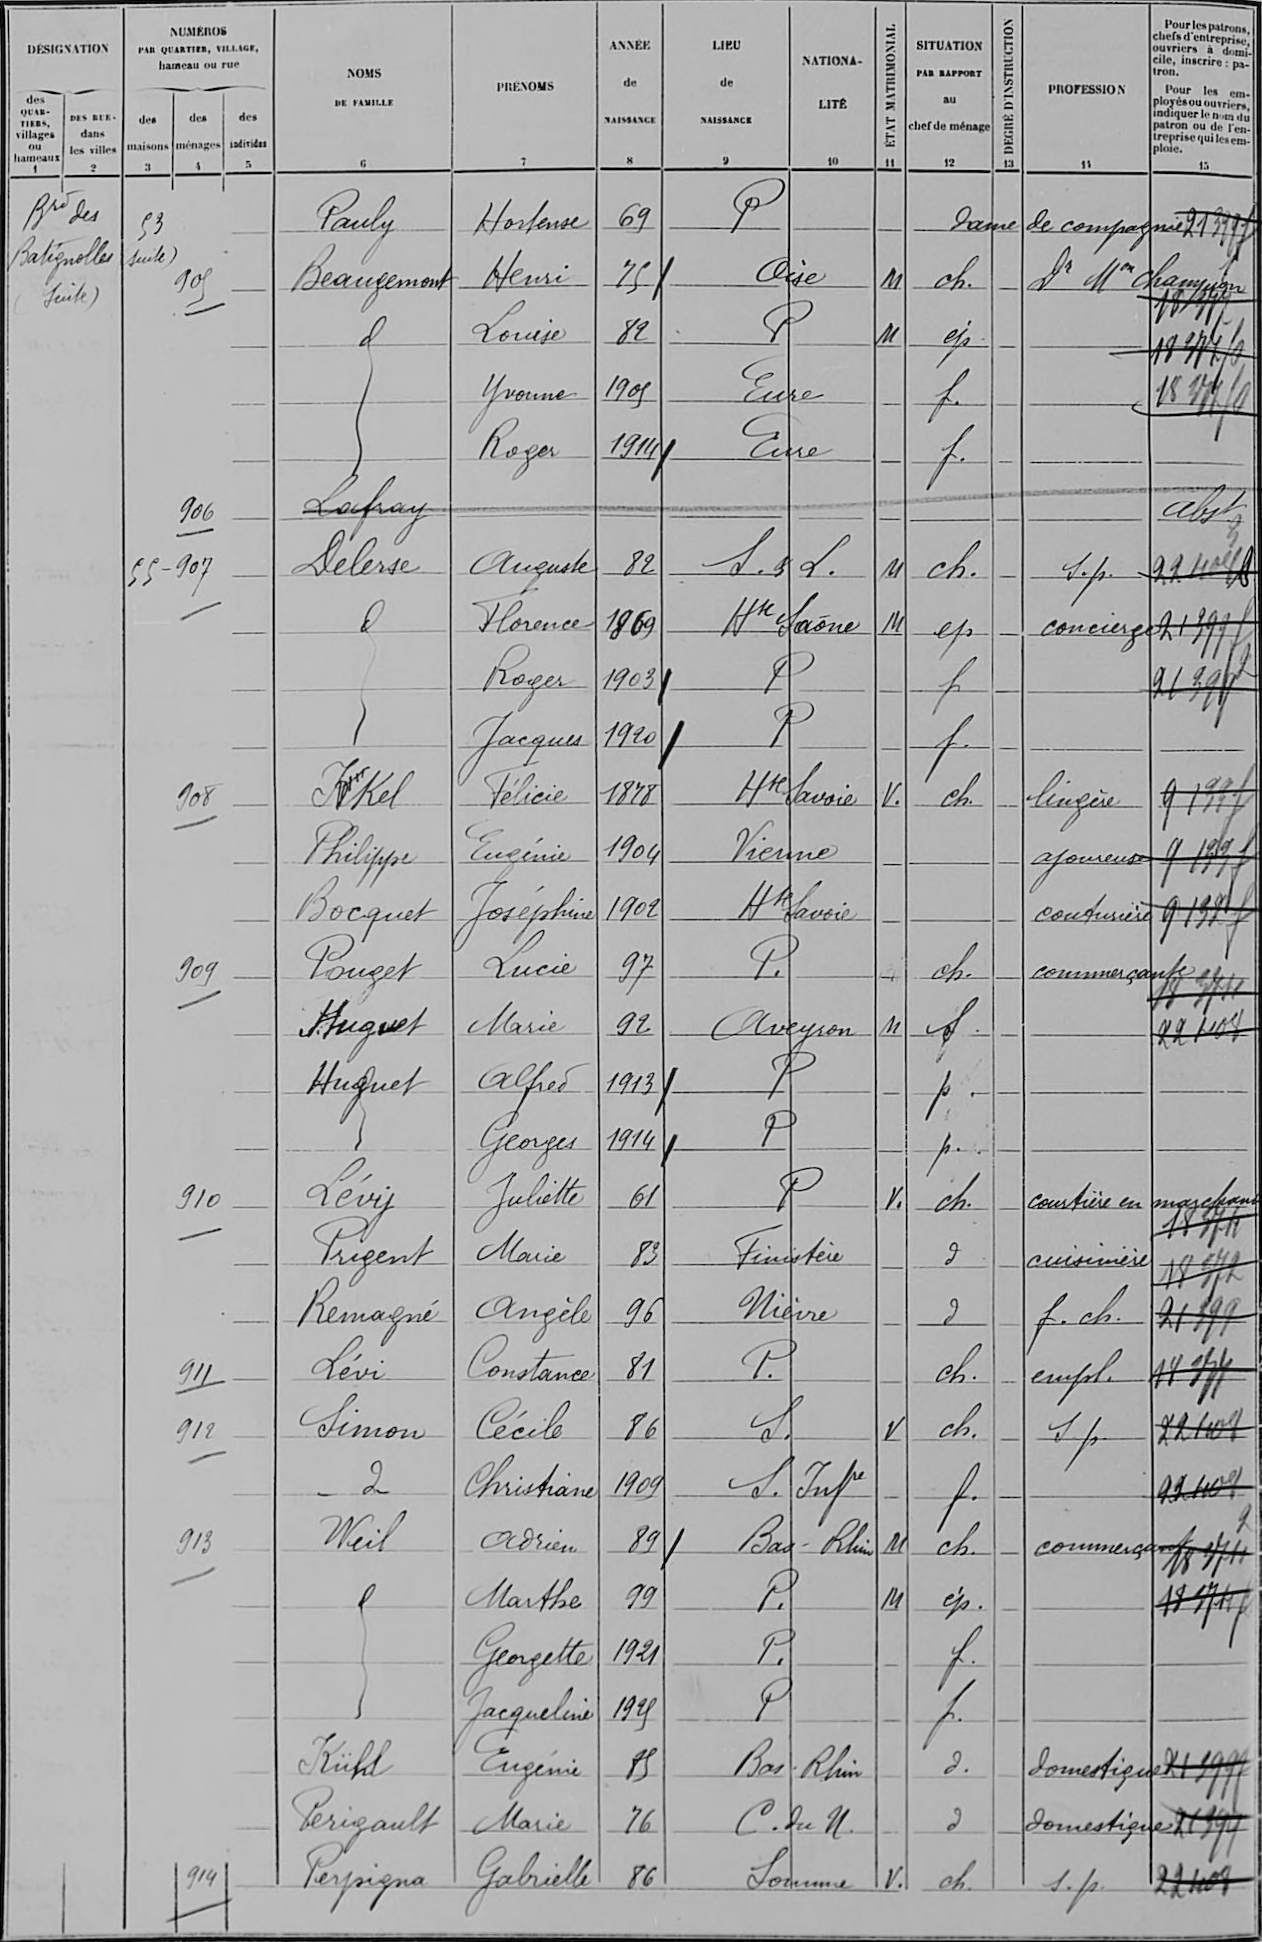Pauly/Hortense/69/P/¤/¤/¤/¤/dame de compagnie/21399 f
Beaugemont/Henri/75/Oise/¤/M/ch /¤/Dr Mon champion/¤
d/Louise/82/P/¤/M/ép/¤/¤/18377 fs
d/Yvonne/1905/Eure/¤/¤/f /¤/¤/18377 fs
d/Roger/1914/Eure/¤/¤/f /¤/¤/¤
Lefray/¤/¤/¤/¤/¤/¤/¤/¤/abs
Delerse/Auguste/82/S & L /¤/M/ch /¤/S p /22408 f
d/Florence/1869/Hte Saône/¤/M/ep/¤/concierge/21399 f
d/Roger/1903/P/¤/¤/f/¤/¤/21399
d/Jacques/1920/P/¤/¤/f /¤/¤/¤
Ikel/Félicie/1878/Hte Savoie/¤/V /ch /¤/lingère/9133 f
Philippe/Eugénie/1904/Vienne/¤/¤/¤/¤/ajoureuse/9133 f
Bocquet/Joséphine/1902/Hte Savoie/¤/¤/¤/¤/couturière/9132 f
Pouget/Lucie/97/P /¤/¤/ch /¤/commerçant/!18374
Huguet/Marie/92/Aveyron/¤/M/f/¤/¤/22408
Huguet/Alfred/1913/P/¤/¤/p /¤/¤/¤
¤/Georges/1914/P/¤/¤/p /¤/¤/¤
Lévy/Juliette/61/P/¤/V /ch /¤/courtière en marchand /!18374
Prigent/Marie/83/Finistère/¤/¤/d/¤/cuisinière/18372
Remagné/Angèle/96/Nièvre/¤/¤/d/¤/f ch /21399
Lévi/Constance/81/P /¤/¤/ch /¤/empl /18377
Simon/Cécile/86/S /¤/V/ch /¤/S p/22408
d°/Chistiane/1909/S Infre/¤/¤/f /¤/¤/22408
Weil/Adrien/89/Bas-Rhin/¤/M/ch /¤/commerçant/18374
d/Marthe/00/P /¤/M/ép/¤/¤/18374
d/Georgette/1921/P /¤/¤/f /¤/¤/¤
d/Jacqueline/1925/P/¤/¤/f /¤/¤/¤
Kühl/Eugénie/85/Bas-Rhin/¤/¤/d /¤/domestique/21399 f
Perigault/Marie/76/C du N /¤/¤/d/¤/domestique/21399
Perpigna/Gabrielle/86/Somme/¤/V /ch /¤/s p /22408
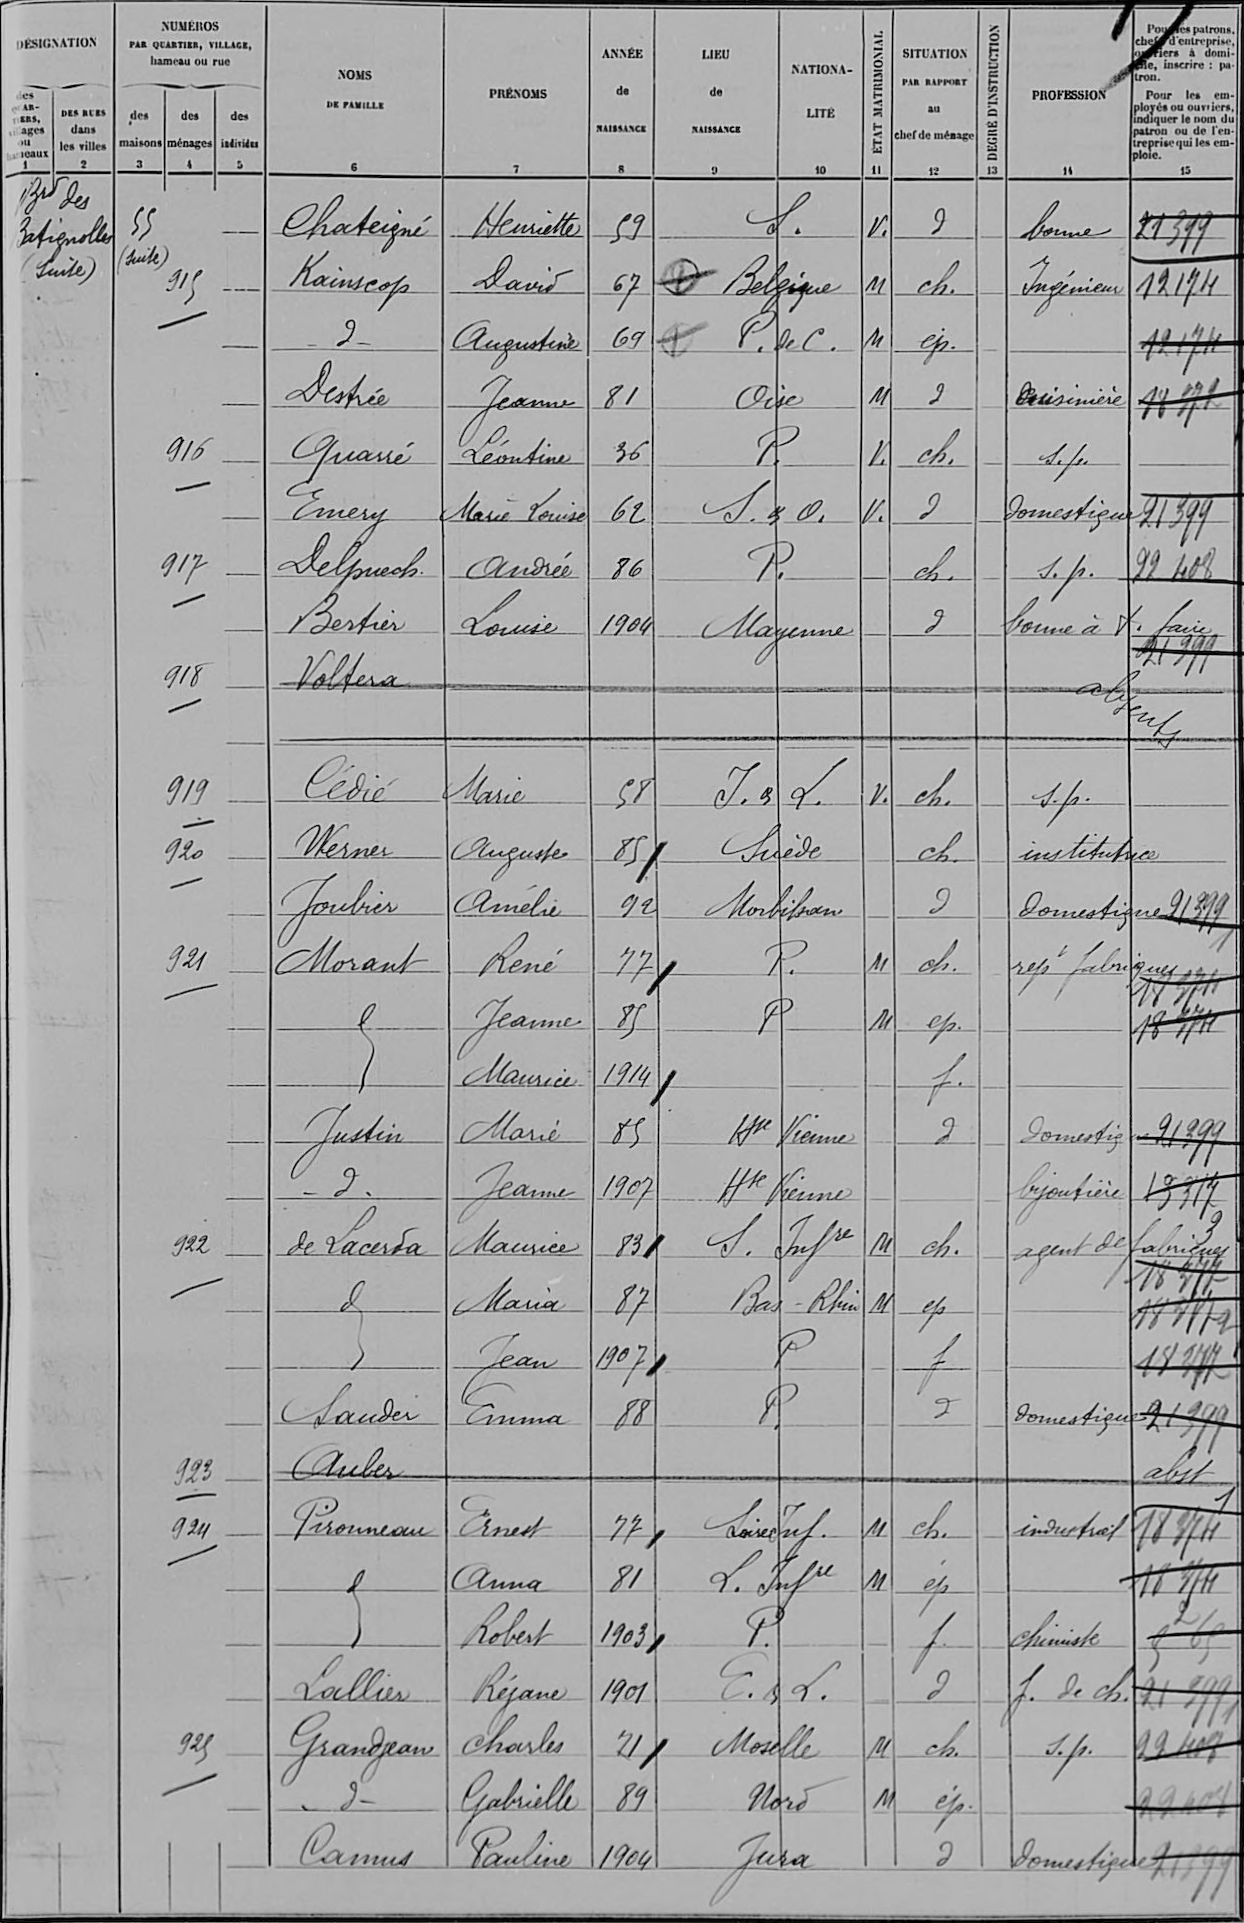Chateigné/Henriette/59/S /¤/V /d/¤/bonne/21399
Kainscop/David/67/Belgique/¤/M/ch /¤/Ingénieur/12174
-d-/Augustine/69/P de C /¤/M/ép /¤/¤/12174
Destrée/Jeanne/81/Oise/¤/M/d/¤/cuisinière/18372
Quarré/Léontine/36/P /¤/V /ch /¤/s p /¤
Emery/Marie Louise/62/S & O /¤/V /d/¤/domestique/21399
Deslpueh/Andrée/86/P /¤/¤/ch /¤/s p /22408
Bertier/Louise/1904/Mayenne/¤/¤/d/¤/bonne à t faire/!21399
Voltera/¤/¤/¤/¤/¤/¤/¤/¤/abs
¤/¤/¤/¤/¤/¤/¤/¤/¤/¤
C2dié/Marie/58/I et L /¤/V /ch /¤/s p /¤
Werner/Auguste/85/Suède/¤/¤/ch /¤/institutrice/¤
Joubier/Amélie/92/Morbihan/¤/¤/d/¤/domestique/21399
Morant/René/77/P /¤/M/ch /¤/rept fabriques/!18374
d/Jeanne/85/P/¤/M/ep /¤/¤/18374
d/Maurice/1914/¤/¤/¤/f /¤/¤/¤
Justin/Marie/85/Hte Vienne/¤/¤/d/¤/domestiq/21399
-d-/Jeanne/1907/Hte Vienne/¤/¤/¤/¤/bijoutière/13317
de Lacerba/Maurice/83/S Infre/¤/M/ch /¤/agent de fabriques/!18377
d/MAria/87/Bas-Rhin/¤/M/ep/¤/¤/18377
d/Jean/1907/P/¤/¤/f/¤/¤/18377
Sauder/Emma/88/P /¤/¤/d/¤/domestique/21399
Auber/¤/¤/¤/¤/¤/¤/¤/¤/abst
Pironneau/Ernest/77/Loire Inf /¤/M/ch /¤/industriel/18374
d/Anna/81/L Infre/¤/M/ép/¤/¤/18374
d/Robert/1903/P /¤/¤/f /¤/chimiste/565
Lallier/Réjane/1901/E & L /¤/¤/d/¤/f de ch /21399
Grandjean/charles 71/Moselle/¤/¤/M/ch /¤/s p /22408
-d-/Gabrielle/89/Nord/¤/M/ép /¤/¤/22408
Camus/Pauline/1904/Jura/¤/¤/d/¤/domestique/21399